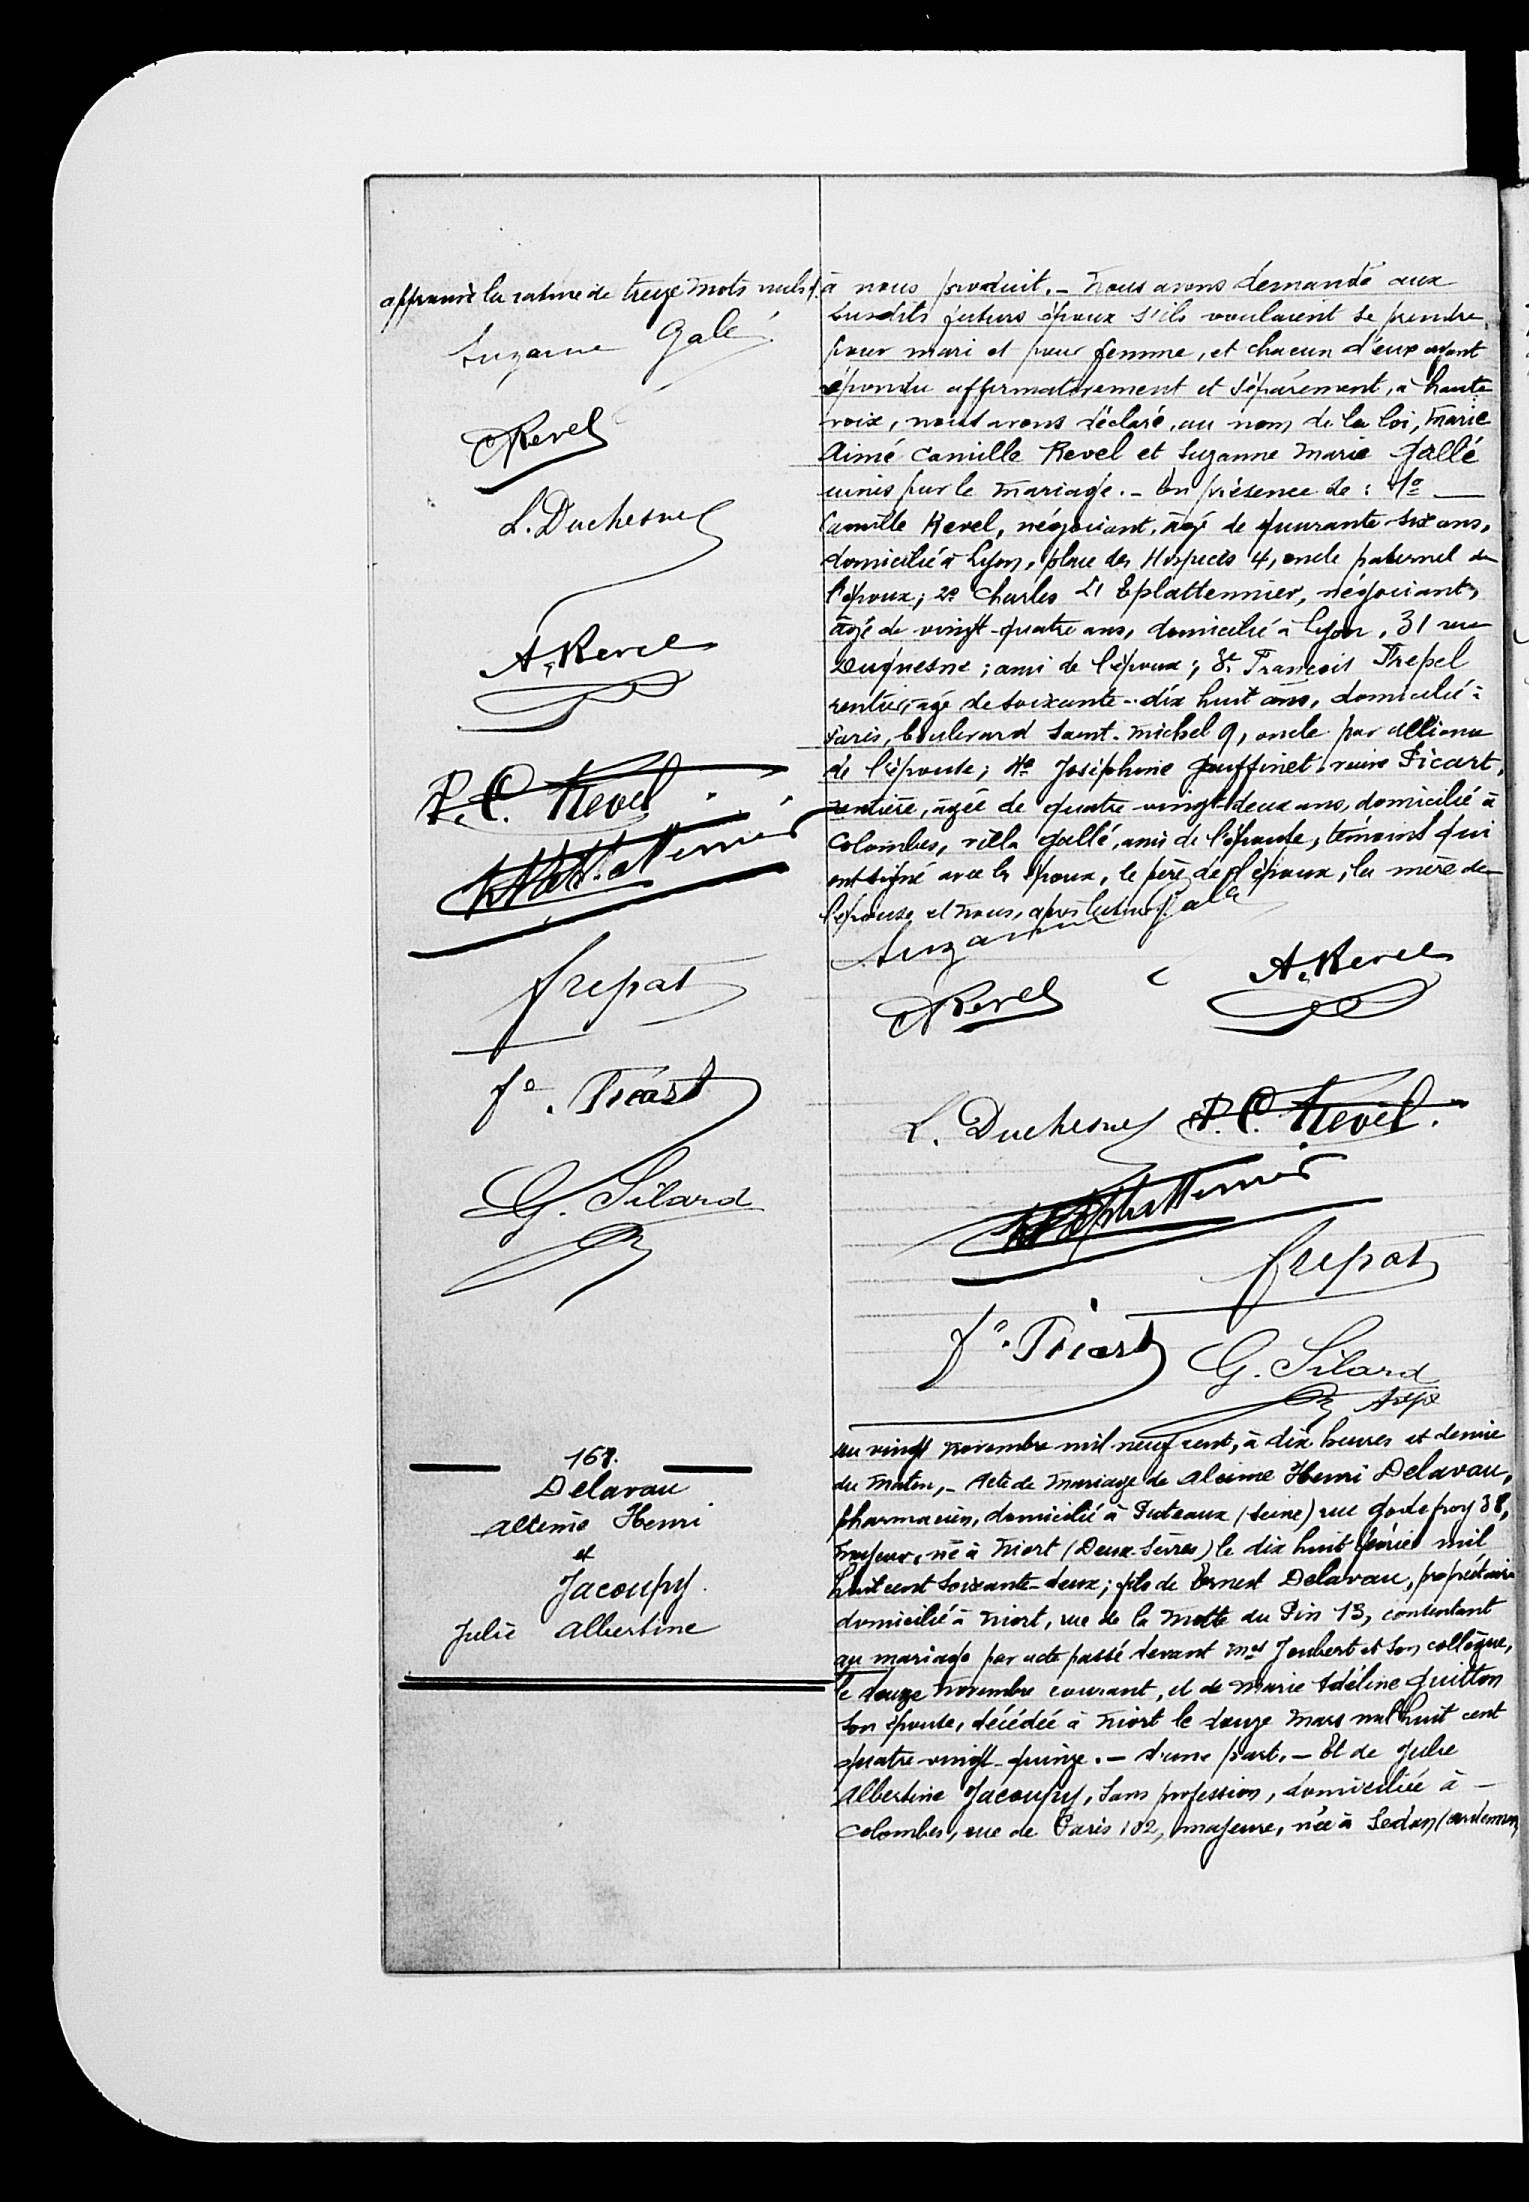à nous produit. Nous avons demandé aux
susdits futurs époux s'ils voulaient se prendre
pour mari et pour femme, et chacun d'eux ayant
répondu affirmativement et séparément, à haute
voix, nous avons déclaré, au nom de la loi, Marie
Aimé, Camille Revel et Suzanne Marie Gallé
unis par le mariage.-en présence de: 1°:-
Camille Kevel, négociant, âgé de quarante six ans,
domicilié à Lyon, place Hospices 5, oncle paternel de
l'époux,; 2°: Charles Eplattemier, négociants
âgé de vingt quatre ans, domicilié à Lyon, 31 rue
Dugnesne; ami de l'épouse; 3° François Frepel
rentier, âgé de soixante-dix huit ans, domicilié à
Paris, boulevard Saint-Michel 9, oncle par alliance
de l'épouse; 4° Joséphine Gouffinet, veuve Picart,
rentière, âgée de quatre-vingt-deux ans, domicilié à
Colombes, villa gallé, ami de l'épouse, témoins qui
ont signé avec les époux, le père de l'époux la mère de
l'épouse et et nous, après lecture
168
Delavou
altème Henri
et
Jacoupy
Julie Albertine
au vingt novembre mil neuf cent, à dix heures et demie
du matin,-Acte de mariage de Alaime Henri Delavau,
pharmacien, domicilié à Puteaux (Seine) rue godefroy 38,
Infeur, né à Niort (Deux-Sèvres) le dix huit février mil
huit cent soixante-deux; fils de Ernest Delavau, propriétaire
domicilié à Niort, rue de la Motte du Pin 13, consentant
au mariage par notre passé devant Ms Joubert et son collègue,
le douze novembre courant, et de Marie Adeline Guitton
son épouse, décédée à Niort le douze mars mil huit cent
quatre-vingt-quinze.-D'une part,-Et de Julie
Albertine Jacoupy, sans profession, domiciliée à-
Colombes, rue de Paris 102, majeure, née à Sedan (ardennes)
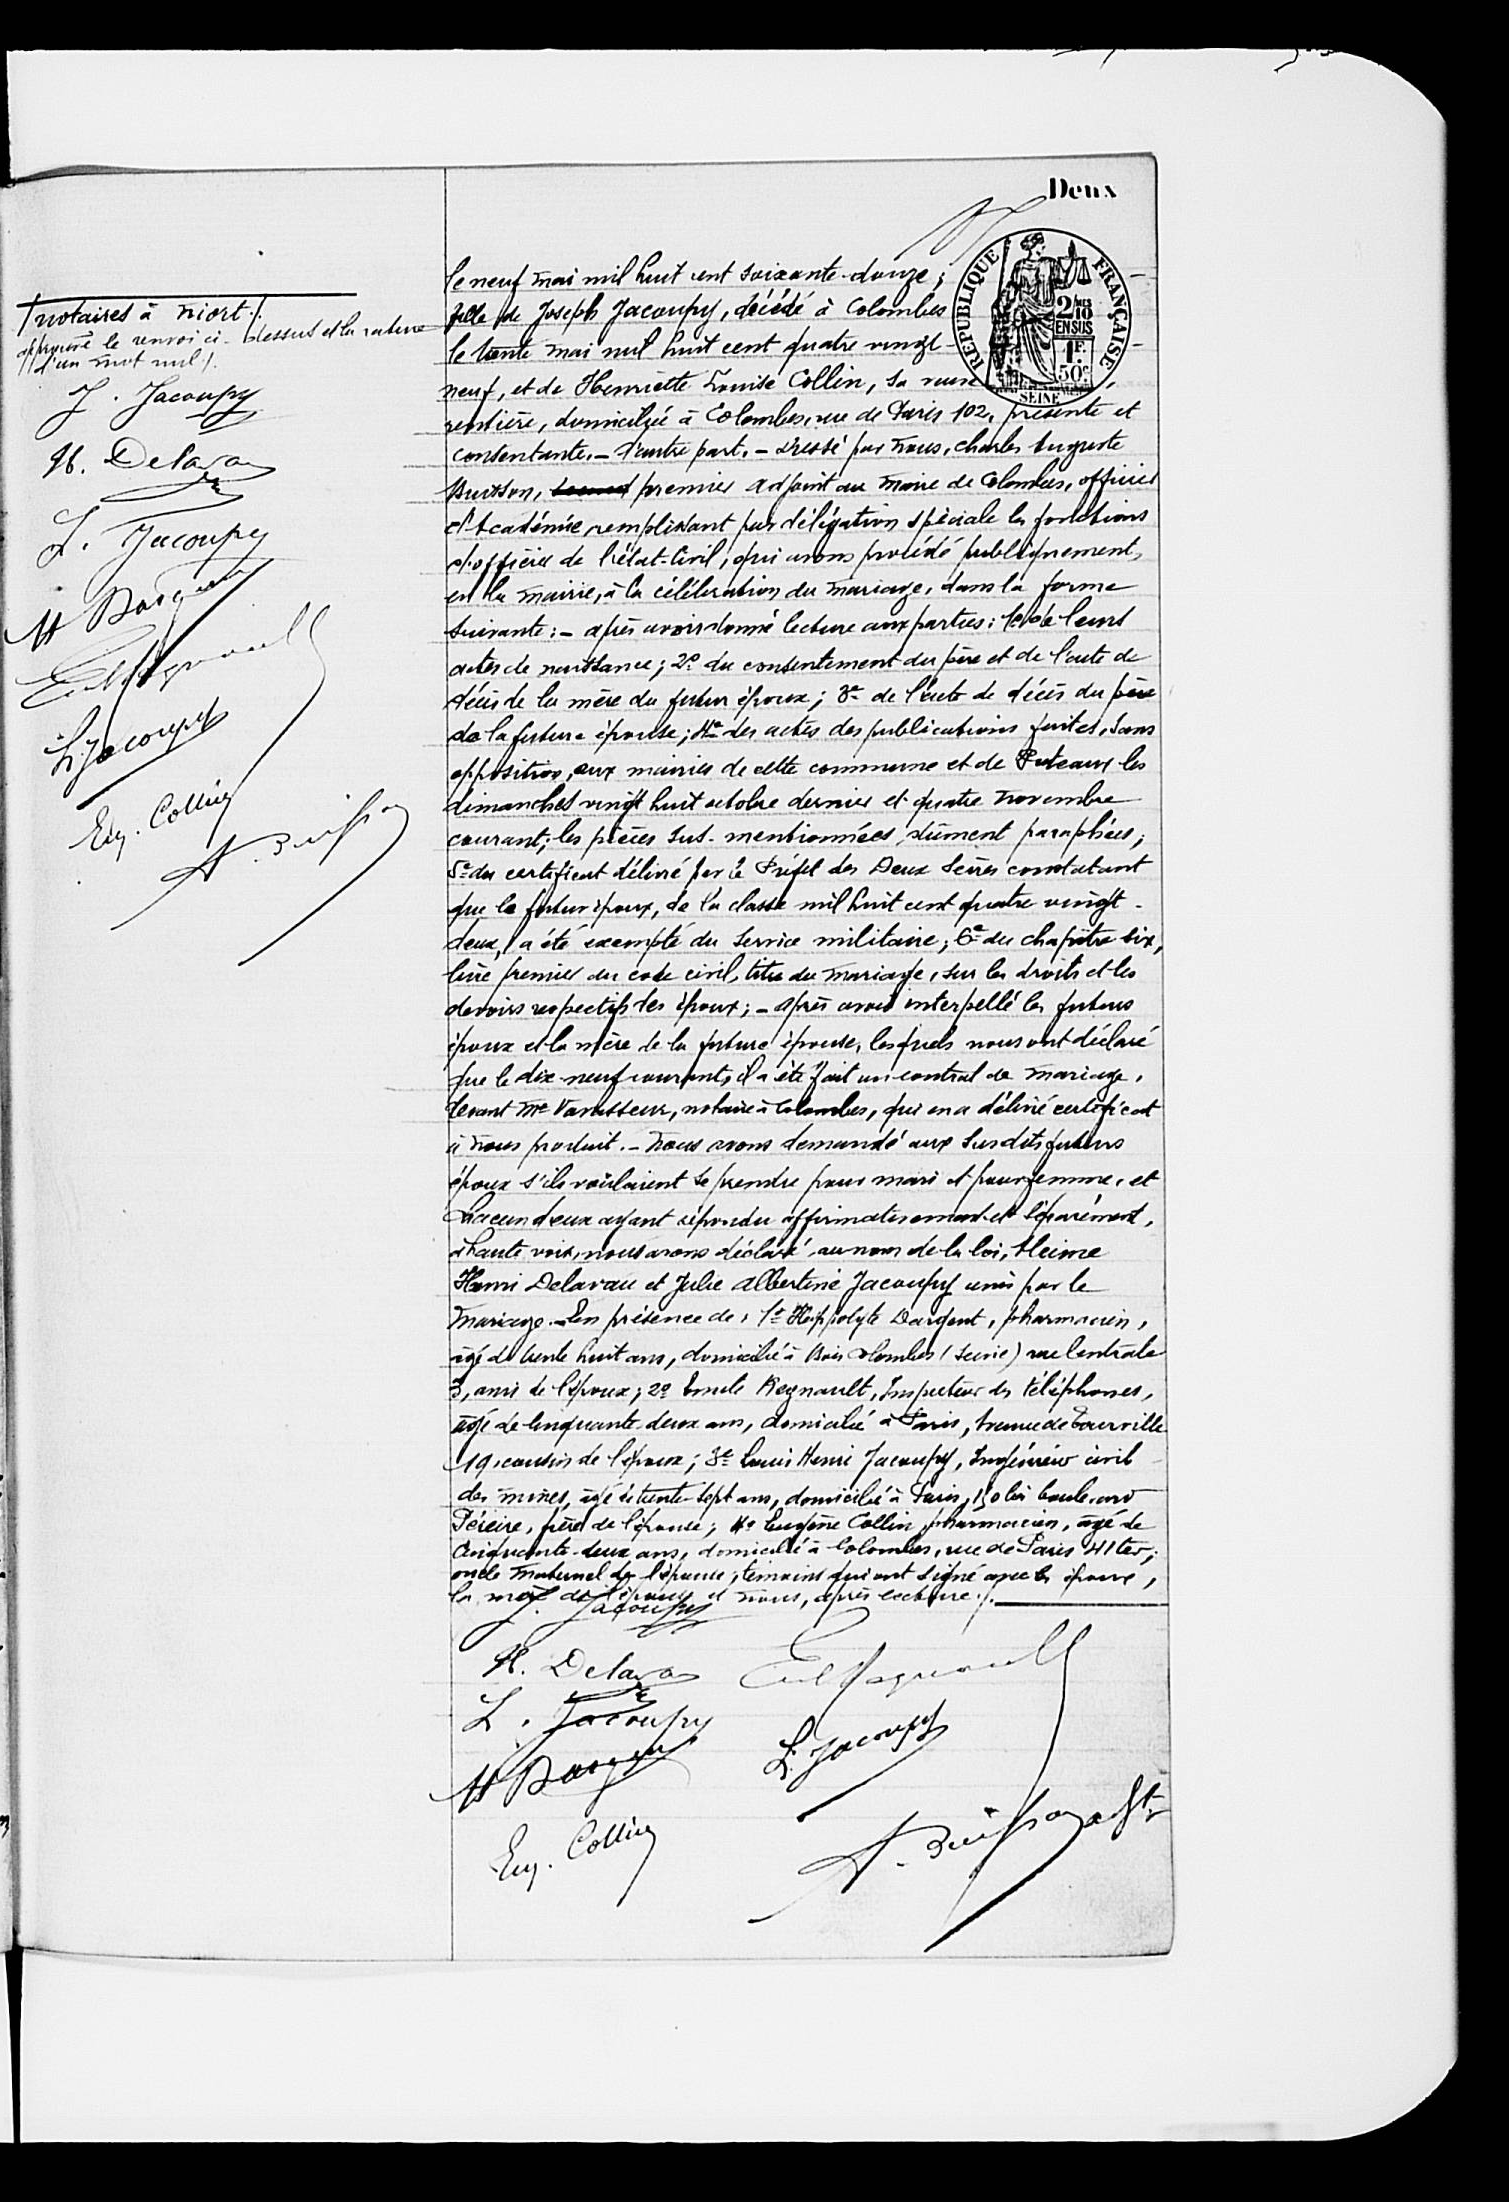le neuf mai mil huit cent soixante-douze;
fille de Joesph Jacoupy, décédé à Colombes
le trente mai mil huit cent quatre vingt-
neuf, et de Henriette Louise Collin, sa veuve
rentière, domiciliée à Colombes, rue de Paris 102, présente et
consentantes.-D'autre part.-Dressé par Nous, Charles, Auguste
Buisson, premier adjoint au Maire de Colombes, officier
d'Académie remplissant par délégation spéciale les fonctions
d'officier de l'état-civil, qui avons procédé publiquement,
en la mairie, à la célébration du mariage, dans la forme
suivante: -après avoir donné lecture aux parties: 1°: de leurs
actes de naissance; 2°: du consentement du père et de l'acte de
décès de la mère du futur époux; 3°: de l'acte de décès du père
de la future épouse; 4°: des actes des publications faites, sans
opposition, aux mairies de cette commune et de Puteaux les
dimanches vingt huit octobre dernier et quatre novembre
courant; les pièces sus-mentionnées, dûment paraphées;
5°: du certificat délivré par le Préfet des Deux Sèvres constatant
que le futur époux, de la classe mil huit cent quatre vingt-
deux, a été exempté du service militaire; 6°: du chapitre six,
livre premier du code civil, titre du mariage, sur les droits et les
devoirs respectifs des époux ;-après avoir interpellé les futurs
époux et la mère de la future épouse, lesquels nous ont déclaré
que le dix-neuf courant, il a été fait un contrat de mariage,
devant Me Vansseur, notaire à Colombes, qui en a délivré certificat
à nous produit. -Nous avons demandé aux susdits futurs
époux s'ils voulaient se prendre pour mari et pour femme, et
chacun d'eux ayant répondu affirmativement et séaprément,
à haute voix, nous avons déclaré, au nom de la loi, Heime
Henri Delavau et Julie Albertine Jacaupy unis par le
mariage. En présence de: 1°: Hippolyte Dargent, pharmacien,
âgé de trente huit ans, domicilié à Bois Colombes (Seine) rue Centrale
3, ami de l'époux; 2°: Emile Regnault Inspecteur des téléphones,
âgé de cinquante-deux ans, domicilié à Paris, avenue de Tourvil
19,cousin de l'épouse; 3°: Louis Henri Jacoupy, ingénieur civil
des mines, âgé de trente sept ans, domicilié à Paris, 150 bis boulevard
Péreire, père de l'épouse; 4°: Eugène Collin, pharmacien âgé de
cinquante-deux ans, domicilié à Colombes, rue de Paris 41ter,
oncle maternel de l'épouse; témoins qui ont signé avec les épouse,
la mère de l'époux et nous, après lecture ./.